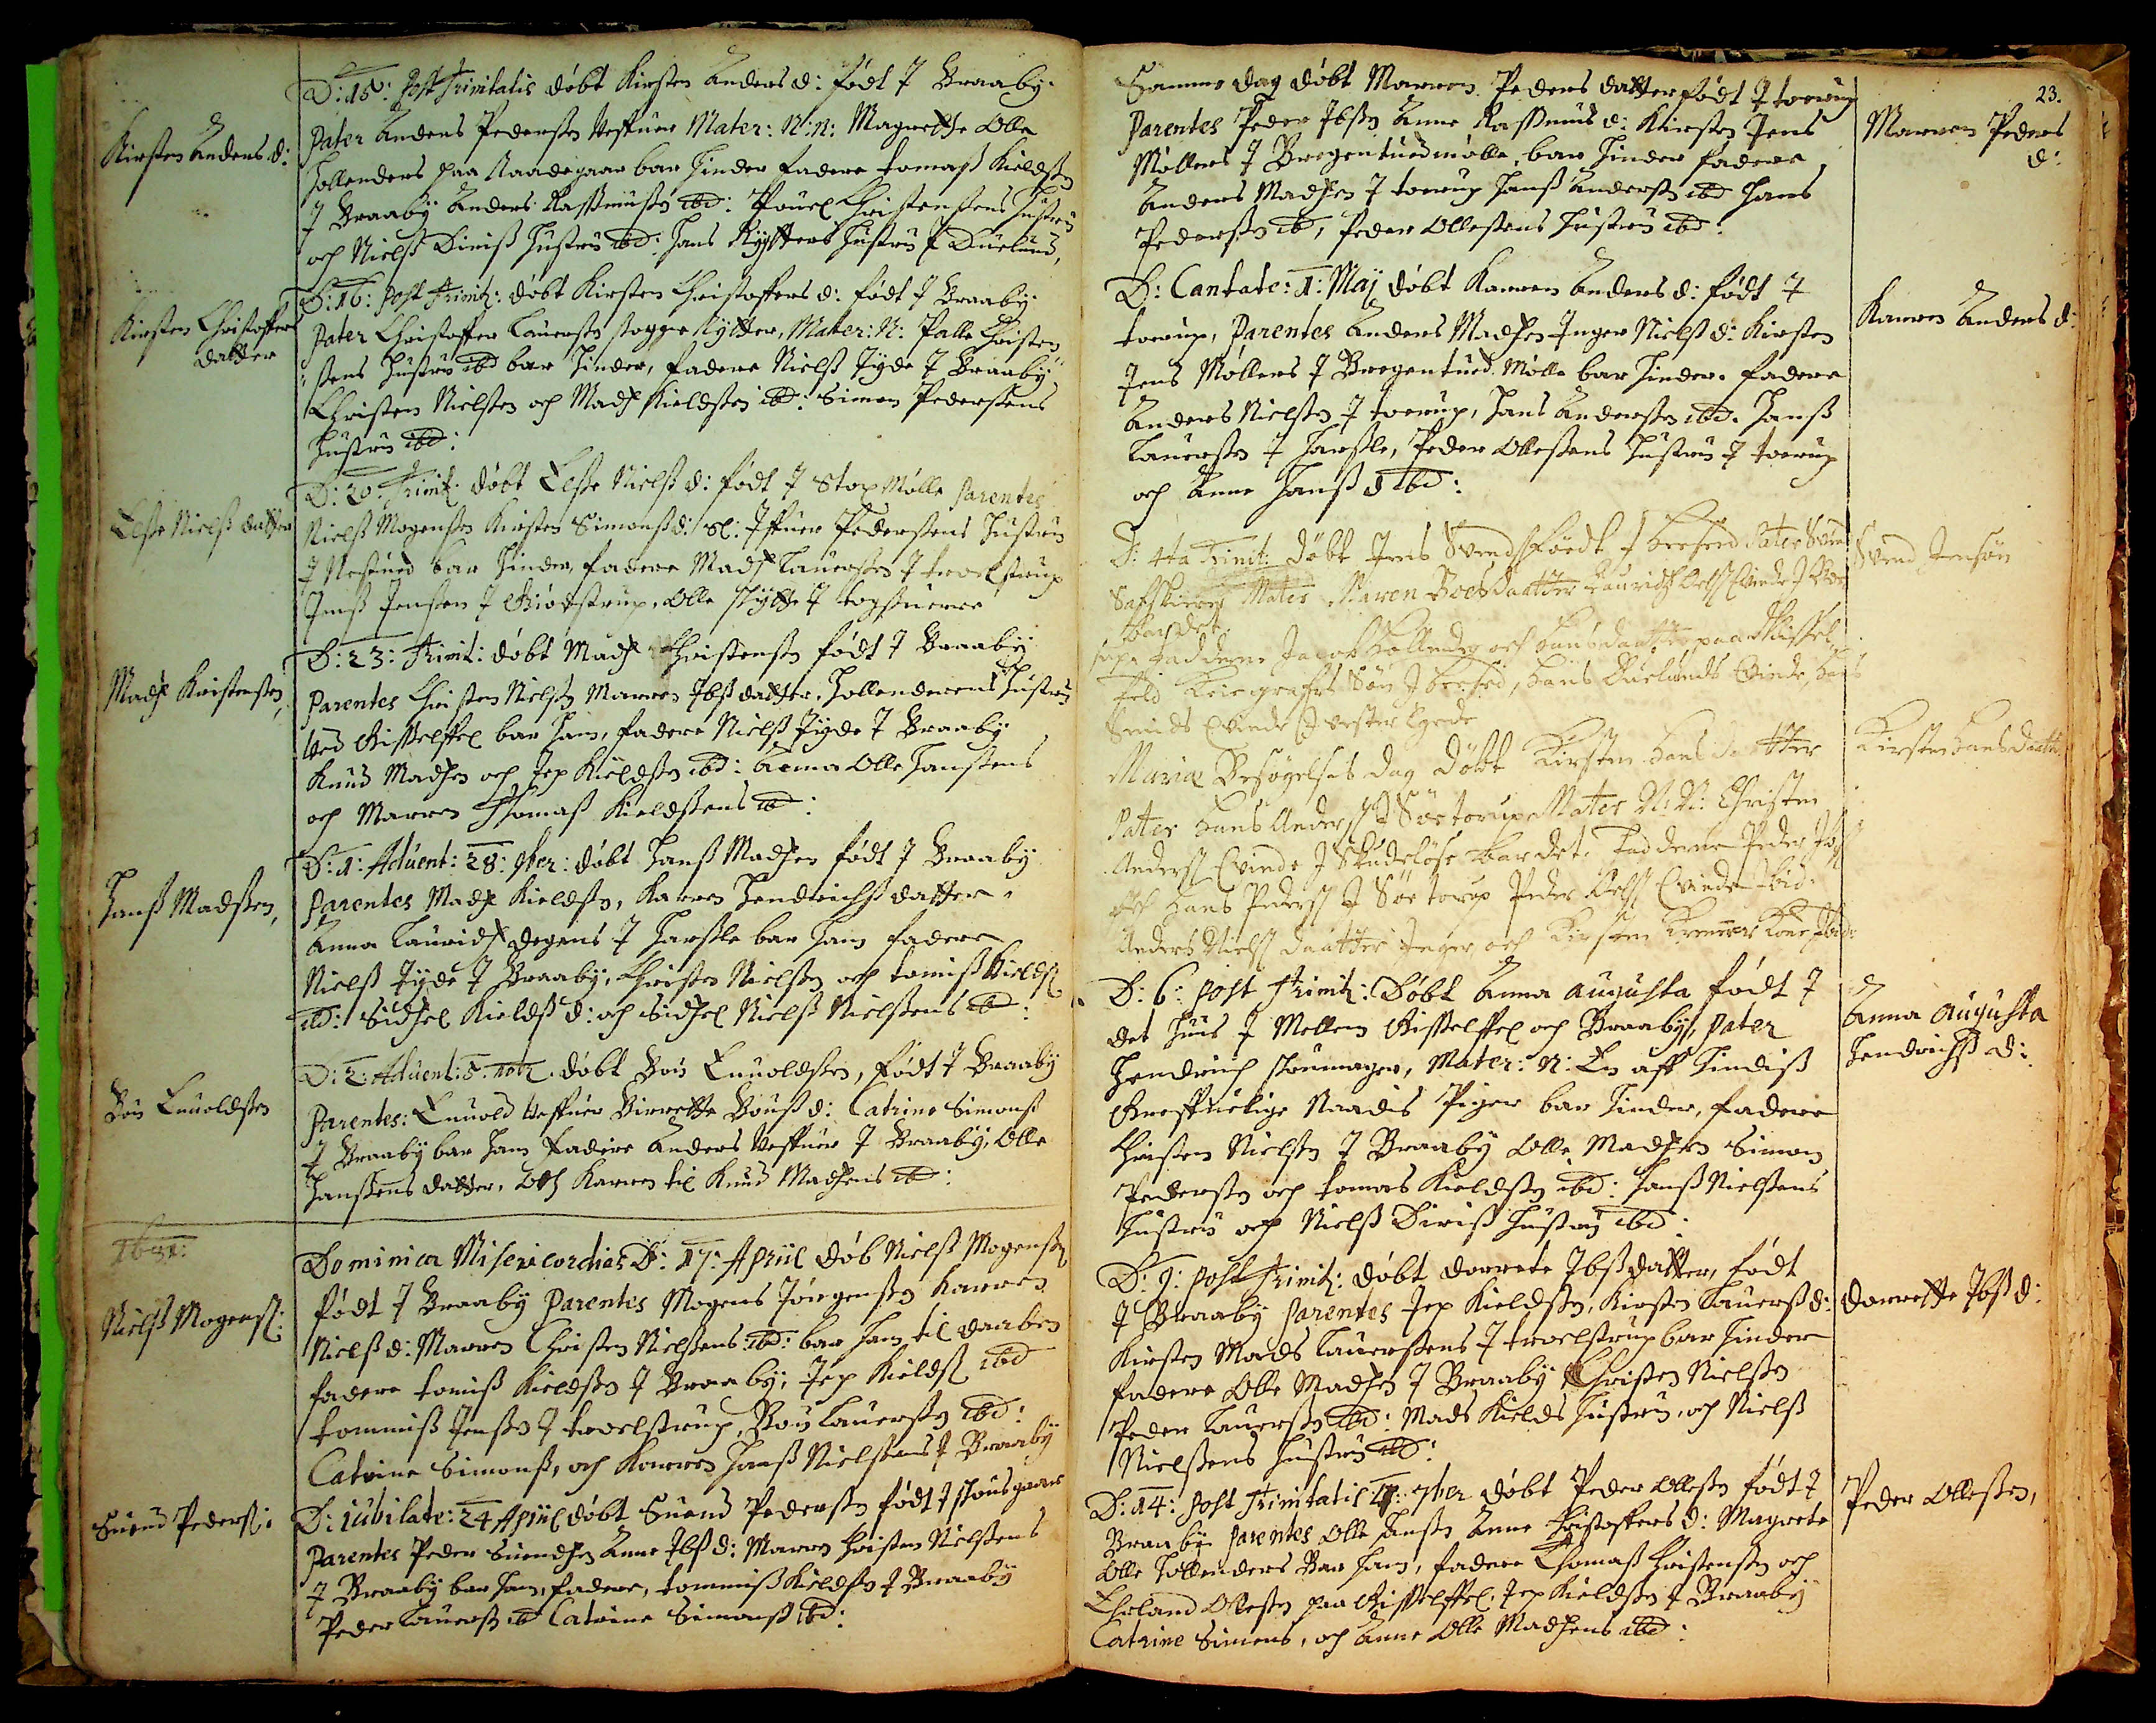Kirsten Anders d: 
D: 15: post Trinitatis døbt Kirsten Anders d: født J Braaby. 
Pater Anders Pedersen Veffuer. Mater: N: N: Magrette Olle 
Hollenders paa Raadegaar bar hinder Fadere Tomas Kields
J Braaby, Anders Rassmusen ibd: Pouel Christensens Hustru
och Niels Diris Hustru ibd: Hans Rytters Hustru J Duelund.
Kirsten Christoffers 
datter 
D: 16: post Trinit: døbt Kirsten Christoffers d: født J Braaby 
Pater Christoffer Lauersen stogge## Rytter, Mater: N: Palle Christen¬
sens Hustru ibd. bar hinder, Fadere Niels Jyde J Braaby.
Christen Nielsen och Mads Kieldsen ibd. Simon Pedersens
Hustru ibd:
Else Niels datter 
D: 20: Trinit. døbt Else Niels d: født J Stox Mølle. Parentes: 
Niels Mogensen Kirsten Simonsd: Sl: Jffuer Pedersens Hustru 
J Nestued bar hinder, Fadere Mads Lauersen J Troelstrup.
Jens Jensen J Giødstrup. Olle Skytte J Togsuerre. 
Mads Kristensen
D: 23: Trinit. døbt Mads Christensen født J Braaby.
Parentes Christen Nielsen, Marren Jbsdatter, Hollenderens Hustru
ved Gisselffel bar ham, Fadere Niels Jyde J Braaby.
Knud Madsen och Jep Kieldsen ibd: Anna Olle Hansens
och Marren Thomas Kieldsens ibd:
Hans Madsen 
D: 1: Aduent: 28: 9ber: døbt Hans Madsen født J Braaby.
Parentes Mads Kieldsen, Karren Hendrichs datter.
Anna Laurids degens J Harsle bar ham Fadere 
Niels Jyde J Braaby, Christen Nielsen och Tomis Kieldsen 
ibd. Sidsel Kields d: och Sidsel Niels Nielsens ibd:
Bou Enuoldsen
D: 2: Aduent: 5. 10br. døbt Bou Enuoldsen, født J Braaby.
Parentes: Enuold Veffuer, Birrette Bous d:, Catrine Simons 
J Braaby bar ham Fadere Anders Veffuer J Braaby, Olle
Hansens datter, Och Karren til Knud Madsen ibd:
1681:
Niels Mogens:
Dominica Misericordias D: 17: Apriil døb Niels Mogensen 
født J Braaby Parentes Mogens Jørgensen, Karren 
Nielsd: Marren Christen Nielsens ibd. bar ham til daaben.
Fadere Tomis Kieldsen J Braaby; Jep Kields ibd.
Tommis Jensen J Troelstrup. Bou Lauersen ibd:
Catrine Simons, och Karren Hans Nielsens J Braaby.
Suend Pedersen 
D: Jubilate: 24 Apriil døbt Suend Pedersen født J Skousgaar. 
Parentes Peder Suendsen Anne Jbs d: Marren Christen Nielsens 
J Braaby bar ham, Fadere Tommis Kieldsen J Braaby. 
Peder Lauers ibd. Catrine Simons ibd:
Samme dag døbt Marren Peders datter født J Toerup
Parentes Peder Jbsen Anne Rassmus d: Kirsten Jens 
Møllers J Bregentuedmølle bar hinder Fadere
Anders Madsen J Toerup Hans Andersen ibd. Hans
Pedersen ibd. Peder Ollesens Hustru ibd.
23.
Marren Peders 
d:
D: Cantate: 1: Maj døbt Karren Anders d: født J 
Toerup, Parentes Anders Madsen, Jnger Niels d: Kirsten
Jens Møller J Bregentued Mølle bar hinder. Fadere
Anders Nielsen J Toerup, Hans Andersen ibd, Hans
Lauersen J Harsle, Peder Ollesens Hustru J Toerup 
och Anne Hans D. ibd:
Karren Anders d:
D: 4ta trinit: døbt Jens Svends føedt i Heeseed Pater Svend
Safskieies Mater Maren Boesdaatter Lauridz Oels Qvinde i Boes##
bar det. 
inpa## Fadderne Jacob Hollender och Bousdatter paa Gissel
feld, Kiergrafes## søn J Heesed, Hans Duelunds Qvinde, Hans
Smids qvinde J Vester Egede.
Svend Jensøn
Mariæ Besøgelsesdag døbt Kirsten Hansdaatter 
Pater Hans Andersen J Søetorup Mater N: N: Christen 
Andersens Qvinde J Skudeløse bar det. Fadderne Peder Jbs 
och Hans Pedersen J Søetorup. Peder Oelsens Qvinde Jbid.
Anders Nielsens daatter Jnger, och Kirsten Kremmers Kone Jbid:
Kirsten Hansdaatter 
D: 6: post Trinit: Døbt Anna Augusta født J 
det Huus J Mellem Gisselfel och Braaby, Pater 
Hendrich skoumager, Mater: N: En aff hindis 
Greffuelige Naadis Piger bar hinder, Fadere 
Christen Nielsen J Braaby, Olle Madsen, Simon
Pedersen och Tomas Kieldsen ibd: Hans Nielsens
Hustru och Niels Diris Hustru ibd:
Anna Augusta 
Hendrichs d:
D: 9: post Trinitz: døbt Dorrette Jbsdatter født 
J Braaby Parentes Jep Kieldsen, Kirsten Lauersd: 
Kirsten Mads Lauersens J Troelstrup bar hinder 
Fadere Olle Madsen J Braaby, Christen Nielsen 
Peder Lauersen ibd: Mads Kields Hustru, och Niels
Nielsens Hustru ibd:
Dorrette Jbs d:
D: 14: post Trinitatis 4: 7ber døbt Peder Ollesen født J
 Braaby Parentes Olle Hansen, Anne Cristoffers d: Magrete
Olle Hollenders Bar ham, Fadere Thomas Christensen och
Ehrland Ollesen paa Gisselffel, Jep Kieldsen J Braaby
Catrine Simons, och Anne Olle Madsens ibd:
Peder Ollesen
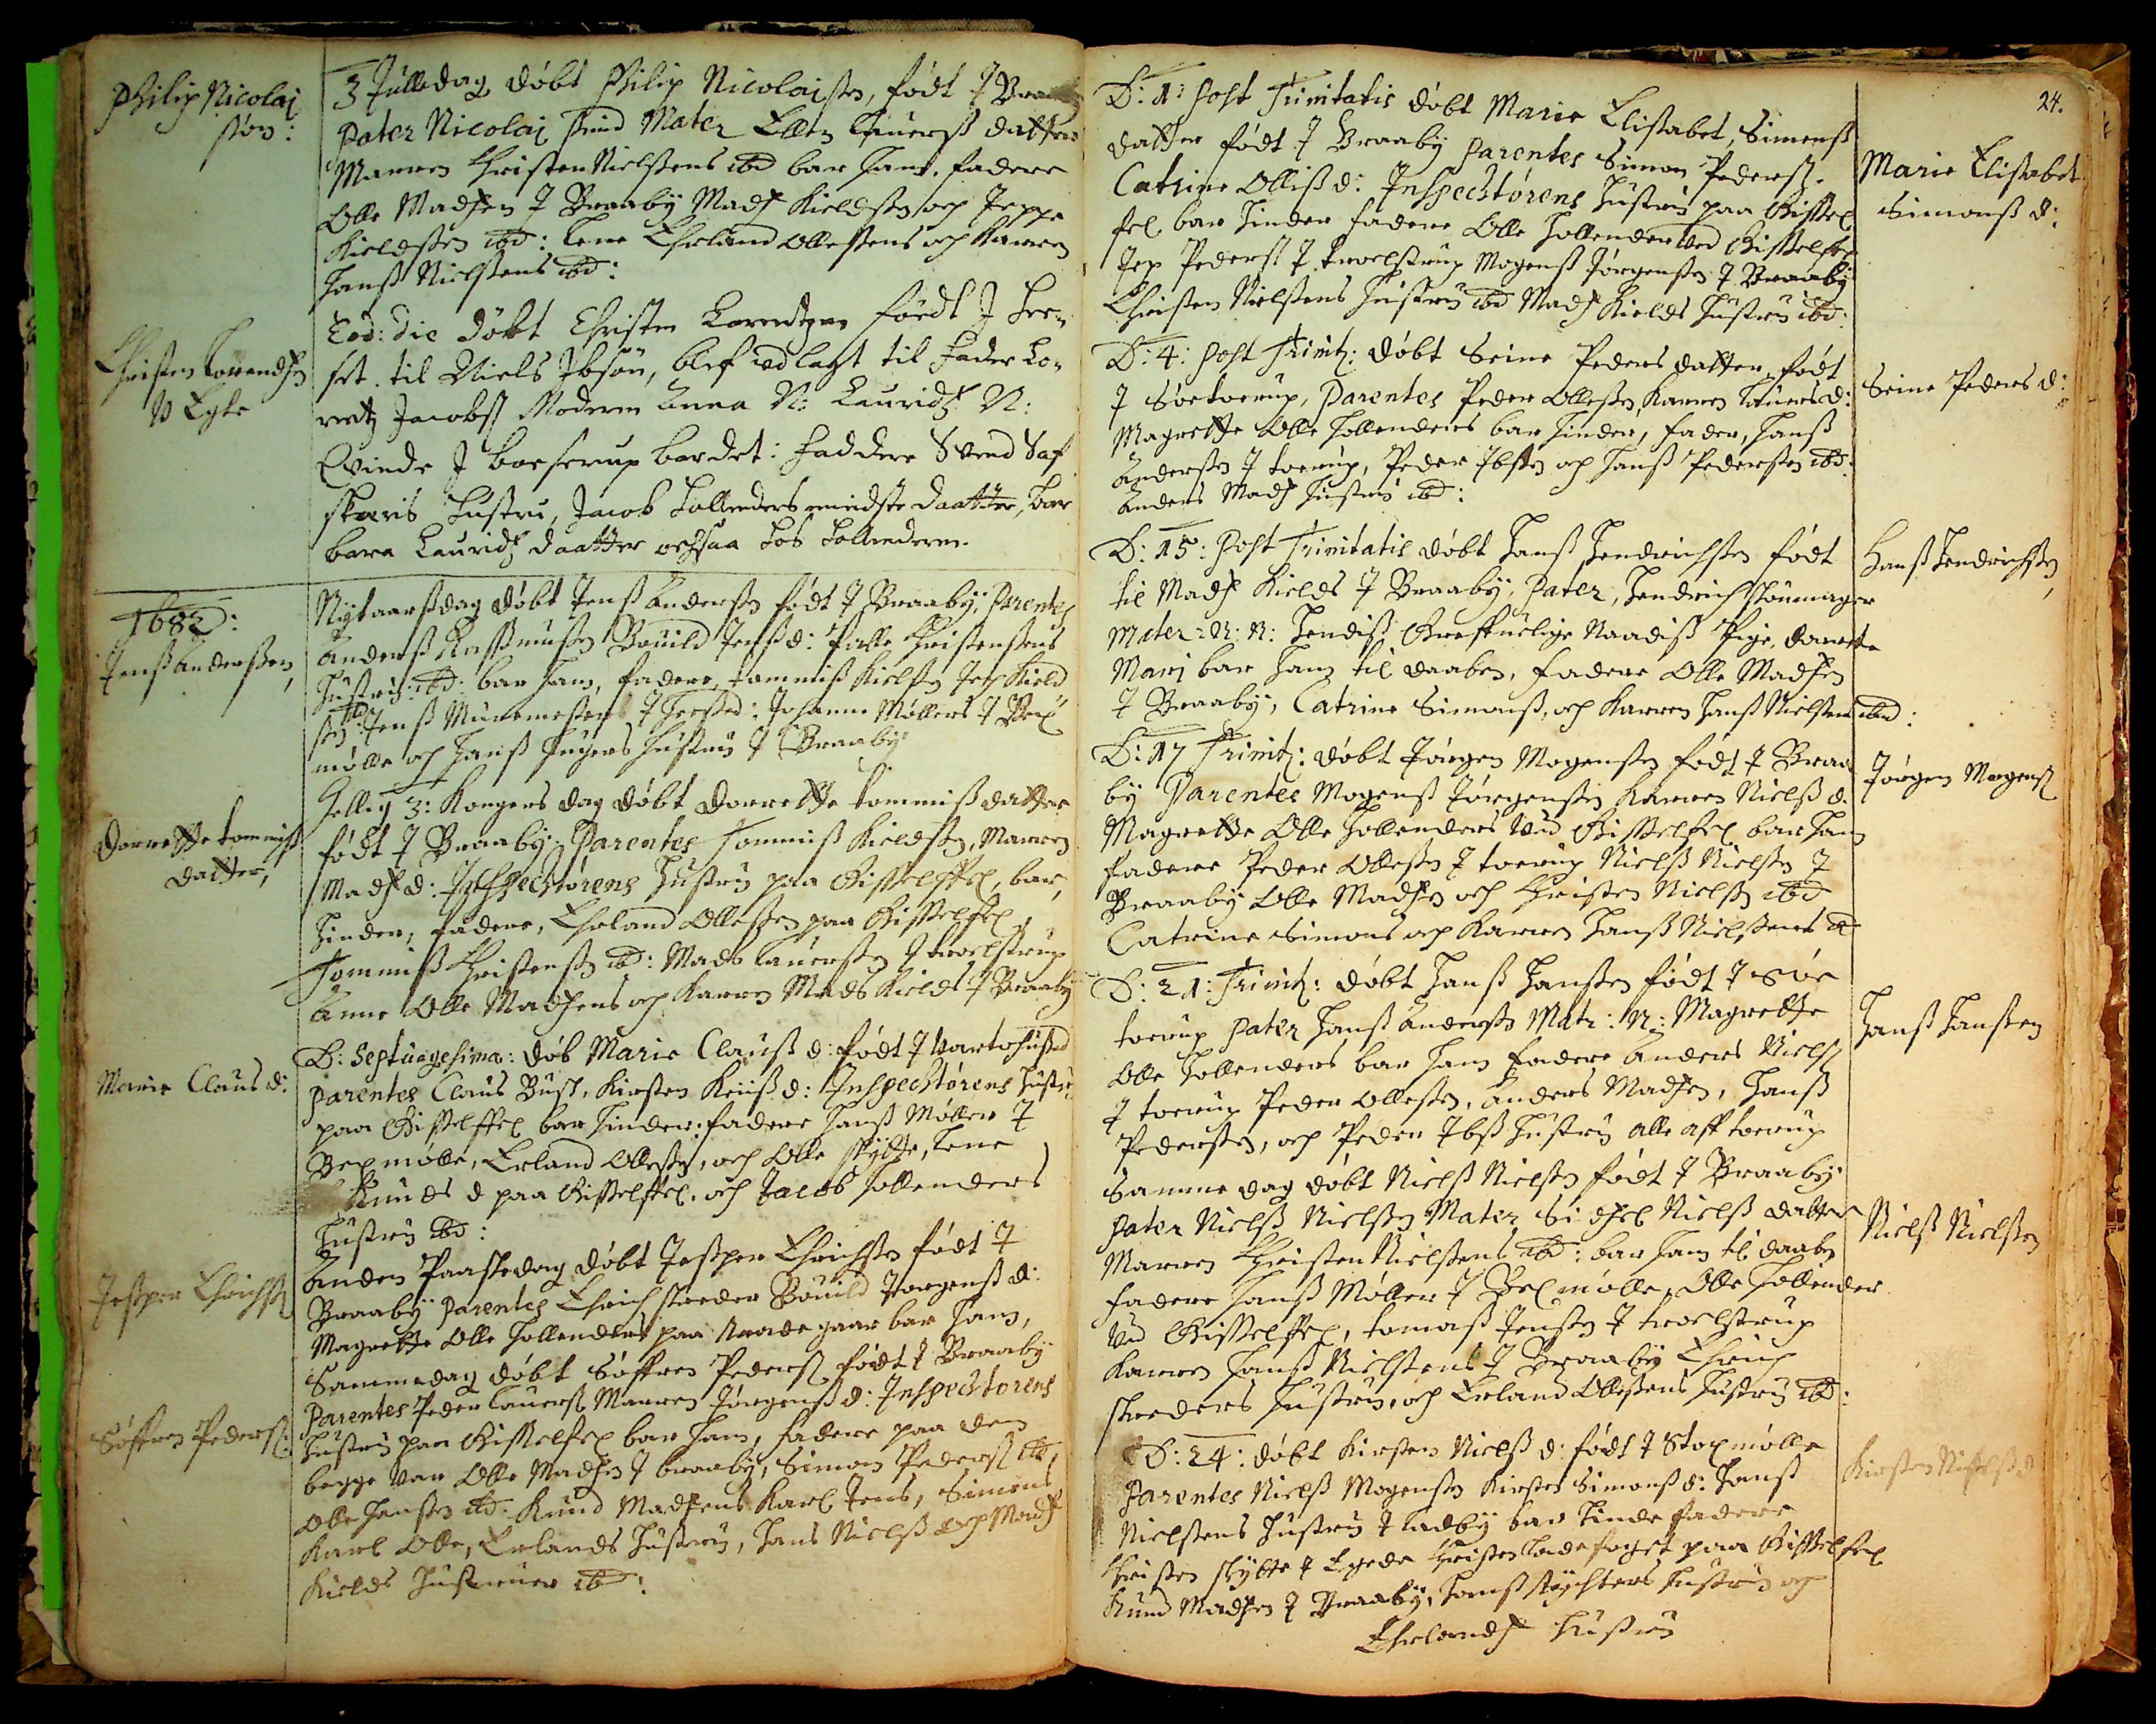Philip Nicolai
søn: 
3 Julledag døbt Philip Nicolaisen født J Braaby. 
Pater Nicolai smid Mater Ellen Lauers datter
Marren Christen Nielsens ibd. bar ham. Fadere
Olle Madsen J Braaby Mads Kieldsen och Jeppe
Kieldsen ibd: Lene Ehrland Ollesen och Karren
Hans Nielsens ibd:
Christen Lorendsen
U Egte 
Eod: Die døbt Christen Lorentzen føedt J Hee¬
set til Niels Jbsøn, blef udlagt til Fader Lo¬
rentz Jacobsen Moderen Anna N: Lauridz N: 
Quinde J Boeserup bar det: Faddere Svend Saf
skæris Hustru, Jacob Hollenders mindste daatter, Bar
bara Laurids daatter ochsaa hos Hollenderen.
1682:
Jens Andersen 
Nytaarsdag døbt Jens Andersen født J Braaby; Parentes: 
Anders Rassmusen, Bouild Jensd: Palle Christensens 
Hustru ibd: bar ham, Fadere Tommis Kieldsen Jep Kield-
ibd
sen: Jens Muremesters J Heesed: Johanne Møllers J Bex
mølle och Hans Hugers Hustru J Braaby: 
Dorrette Tommis 
datter 
hellig 3 kongers dag døbt Dorrette Tommis datter 
født J Braaby. Parentes Tommis Kieldsen, Marren 
Madsd: Inspechtørens Hustru paa Gisselffel, bar,
hinder, Fadere, Ehrland Ollesen paa Gisselfel, 
Tommis Christensen ibd: Mads Lauersen J Troelstrup,
Anne Olle Madsens och Karren Mads Kields J Braaby
Marie Claus d: 
D: Septuagesima: døb Marie Claus d: født J Vartohused
Parentes Claus Busk, Kirsten Keiis d: Inspecktørens Hustru
paa Gisselffel bar hinder: Fadere: Hans Møller J
Bexmølle, Erland Ollesen, och Olle Skytte. Lene
##knuds d paa Gisselffel. och Jacobs Hollenders
Hustru ibd:
Jesper Ehrichsen 
Anden Paaskedag døbt Jesper Ehrichsen født J 
Braaby Parentes Ehrich Skreder Bouild Jørgensens d: 
Magrette Olle Hollenders paa Raadegaar bar ham,
Søffren Pedersen 
Samme dag døbt Søffren Peders født J Braaby.
Parentes Peder Lauersen Marren Jørgensd:. Inspecktorens 
Hustru paa Gisselfel bar ham, Fadere paa dem 
begge var Olle Madsen J Braaby, Simon Pederss ibd.
Olle Jensen ibd. Knud Madsens karl Jens, Simons
karl Olle, Erlands Hustru, Hans Nielsen och Mads
Kields Hustruer ibd:
D: 1: post Trinitatis døbt Marie Elisabet Simons 
datter født J Braaby Parentes Simon Peders
Catrine Ollis d: Inspecktørens Hustru paa Gissel
fel bar hinder. Fadere Olle Hollender ved Gisselfel
Jep Peders J Troelstrup Mogens Jørgensen J Braaby
Christen Nielsens Hustru ibd. Madz Kields Hustru ibd:
24.
Marie Elisabet 
Simons d:
D: 4: post Trinit:  døbt Seine Peders datter født
J Søetoerup, Parentes Peder Ollesen, Karren Lauersd:
Magrette Olle Hollenders bar hinder, Fader, Hans
Andersen J Toerup, Peder Jbsen och Hans Pedersens ibd:
Anders Mads Hustru ibd:
Seine Peders d:
D: 15: Post Trinitatis døbt Hans Hendrichsen født 
til Mads Kields J Braaby, Pater, Hendrich Skoumager.
Mater: N: N: Hendis Greffuelige Naadis Pige, Dorette
Mari bar ham til daaben, Fadere Olle Madsen
J Braaby, Catrine Simons, och Karren Hans Nielsens ibd:
Hans Hendrichsen 
D: 17: Trinit: døbt Jørgen Mogensen født J Braa
by. Parentes Mogens Jørgensen Karren Niels d.
Magrette Olle Hollenders ved Gisselfel bar ham
Fadere Peder Ollesen J Toerup Niels Nielsen J
Braaby, Olle Madsen och Christen Nielsen ibd
Catrine Simons och Karren Hans Nielsens ibd
Jørgen Mogens 
D: 21: Trinit: døbt Hans Hansen født J Søe
toerup. Pater Hans Andersen Mater: N: Magrette 
Olle Hollenders bar ham, Fadere Anders Niels 
J Toerup, Peder Ollesen, Anders Madsen, Hans
Pedersen och Peder Jbsens Hustru alle aff Toerup.
Hans Hansen
Samme dag døbt Niels Nielsen født J Braaby
Pater: Niels Nielsen Mater Sidsel Niels datter
Marren Christen Nielsens ibd. bar ham til daaben
Fadere Hans Møller J Bexmølle, Olle Hollender
ved Gisselffel, Tomas Jensen J Troelstrup
Karren Hans Nielsens J Braaby, Ehrich
Skreders Hustru, och Erland Ollesens Hustru ibd:
Niels Nielsen
D: 24: døbt Kirsten Nielsd: født J Stoxmølle 
Parentes Niels Mogensen, Kirsten Simonsd: Hans 
Nielsens Hustru J Ladby bar hinder, Fadere 
Christen Skytte J Egede, Christen Ladefoget paa Gisselfel,
Knud Madsen J Braaby, Hans Rychters Hustru och
Ehrlands Hustru
Kirsten Niels d: